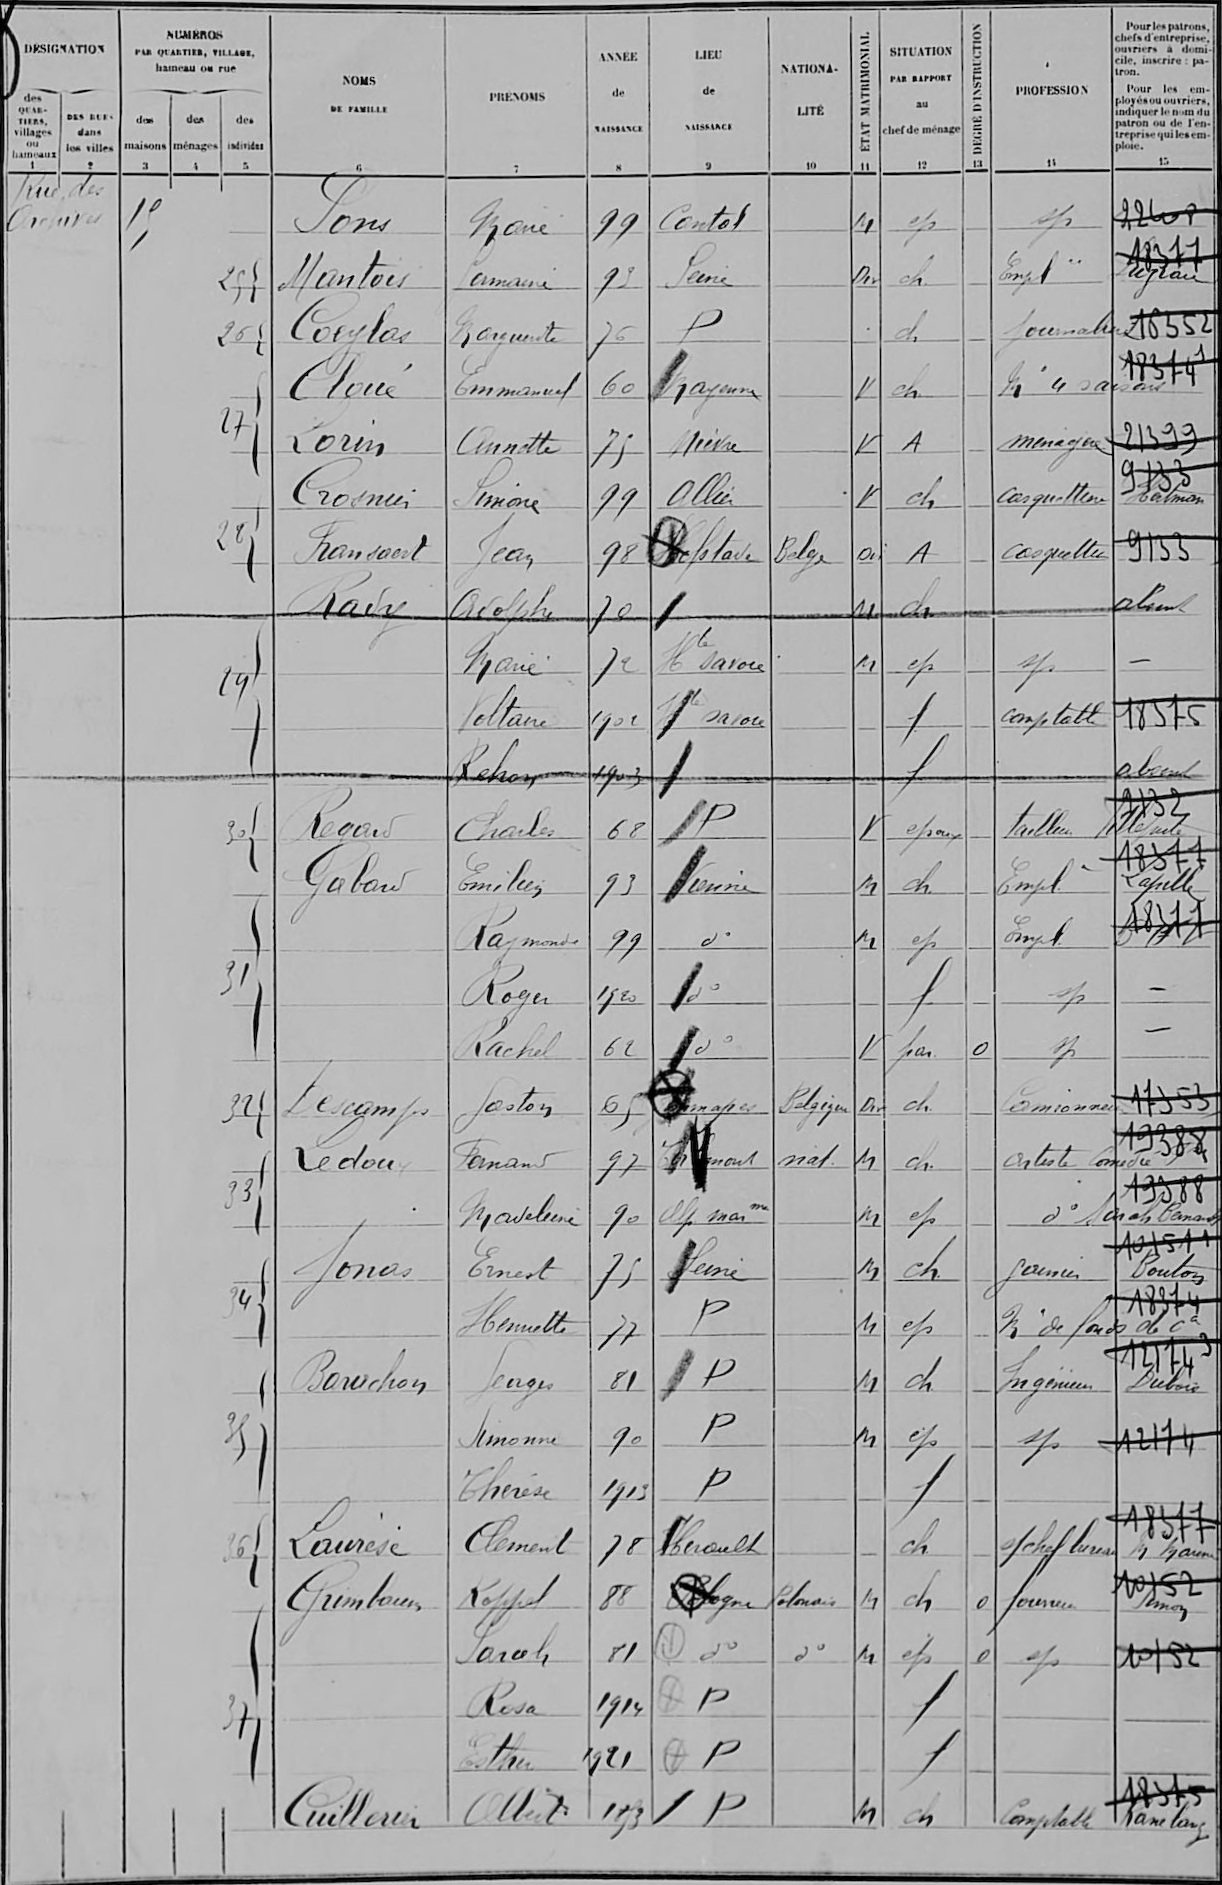Lons/Marie/99/Cantal/¤/M/ep/¤/sp/22408
Mantois/Germaine/93/Seine/¤/Div/ch /¤/Emplee/Aiglan?18377
Coeylas/Marguerite/76/P/¤/¤/ch/¤/Journalière/16352
Cloué/Emmanuel/60/Mayenne/¤/V/Ch/¤/Me 4 saisons/?18374
Lorin/Annette/75/Nièvre/¤/V/A/¤/ménagère/21399
Crosnier/Simone/99/Allier/¤/V/ch/¤/casquetteur/Hatman?9133
Fransaert/Jean/98/Hefstave/Belge/Div/A/¤/casquettier/9133
Rady/Adolphe/70/¤/¤/M/ch/¤/¤/absent
¤/Marie/72/Hte savoie/¤/M/ep/¤/sp/¤
¤/Voltaire/1902/Hte savoie/¤/¤/f/¤/comptable/18375
¤/Rehon/1903/¤/¤/¤/f/¤/¤/absent
Regard/Charles/68/P/¤/V/époux/¤/tailleur/Lillefaite?9132
Gabard/Emilien/93/Vienne/¤/M/ch /¤/Emplé/Lapelle?18377
¤/Raymonde/99/d°/¤/M/ep/¤/Empl /BHL?18377
¤/Roger/1920/d°/¤/¤/f/¤/sp/¤
¤/Rachel/62/d°/¤/V/par/0/p/¤
Lescamps/Gaston/65/Jemapes/Belgique/Div/ch /¤/Camionneur/17353
Ledoux/Fernand/97/C du nord/nat /M/ch /¤/artiste Comédie/?19388
¤/Madeleine/90/Alp Marme/¤/M/ep/¤/d°/Sarah Bernard?19388
Jonas/Ernest/75/Seine/¤/M/ch/¤/gainier/Bouton?10151
¤/Henriette/77/P/¤/M/ep/¤/me de fonds de Cce/?18374
Baruchon/Georges/81/P/¤/M/ch/¤/Ingénieur/Dubois?12174
¤/Simonne/90/P/¤/M/ep/¤/sp/12174
¤/Thérèse/1913/P/¤/¤/f/¤/¤/¤
Laurèse/Clément/78/Hérault/¤/¤/ch/¤/O- chef bureau de mairie/?18377
Grimbaun/Koppel/88/Pologne/Polonais/M/ch/0/fourreur/Simon?10152
¤/Sarah/81/d°/d°/M/ep/0/sp/10152
¤/Rosa/1914/P/¤/¤/f/¤/¤/¤
¤/Esther/1921/P/¤/¤/f/¤/¤/¤
Cuillerier/Albert/1853/P/¤/M/ch/¤/Comptable/Ranelang?18375
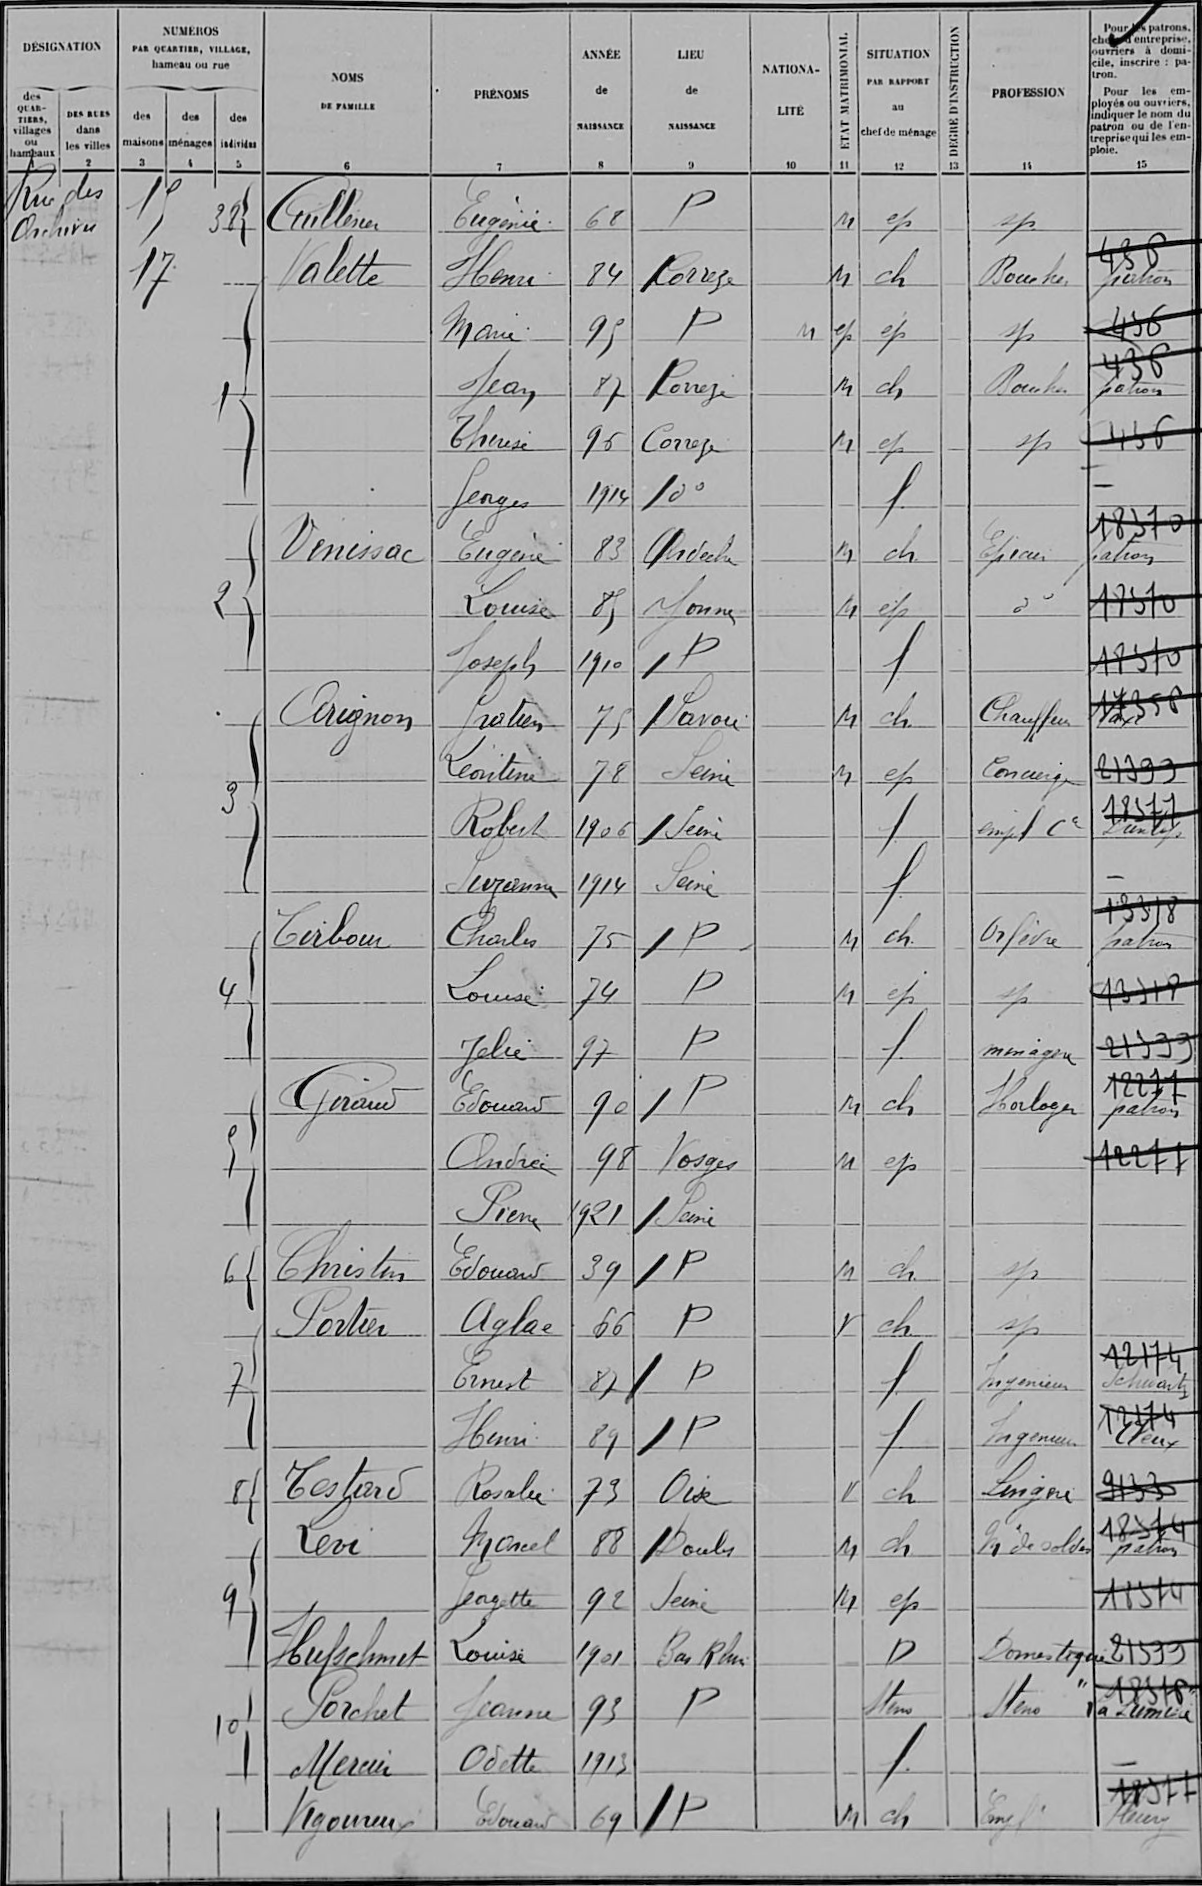Cuillener/Eugénie/68/P/¤/M/ep/¤/sp/¤
Valette/Henri/84/Correze/¤/M/ch/¤/Boucher/patron?436
¤/Marie/95/P/¤/M/ep/¤/sp/436
¤/Jean/87/Correze/¤/M/ch/¤/Boucher/patron?436
¤/Therese/95/Correze/¤/M/ep/¤/sp/436
¤/Georges/1914/d°/¤/¤/f/¤/¤/¤
Venissac/Eugène/83/Ardèche/¤/M/ch/¤/Epicier/patron?18370
¤/Louise/85/Yonne/¤/M/ep/¤/d°/18370
¤/Joseph/1910/P/¤/¤/f/¤/¤/18370
Arignon/Gratien/75/Savoie/¤/M/ch/¤/Chauffeur taxi/?17356
¤/Léontine/78/Seine/¤/M/ep/¤/Concierge/21399
¤/Robert/1906/Seine/¤/¤/f/¤/empl Cce/Dunlop?18377
¤/Suzanne/1914/Seine/¤/¤/f/¤/¤/¤
Tirbour/Charles/75/P/¤/M/ch/¤/Orfèvre/patron?13318
¤/Louise/74/P/¤/M/ep/¤/sp/13318
¤/Zelie/97/P/¤/¤/f/¤/ménagère/21399
Giraud/Edouard/90/P/¤/M/ch/¤/Horloger/patron?12277
¤/Andrée/98/Vosges/¤/M/ep/¤/¤/12277
¤/Pierre/1921/Seine/¤/¤/¤/¤/¤/¤
Christin/Edouard/39/P/¤/M/ch/¤/sp/¤
Portier/Aglae/66/P/¤/V/ch/¤/sp/¤
¤/Ernest/87/P/¤/¤/f/¤/Ingénieur/Schwartz?12174
¤/Henri/89/P/¤/¤/f/¤/Ingénieur/Cleux?12174
Testard/Rosalie/73/Oise/¤/V/ch/¤/Lingère/9133
Levi/Marcel/88/Doubs/¤/M/ch/¤/M de soldes/patron?18374
¤/Georgette/92/Seine/¤/M/ep/¤/¤/18374
Hufschmit/Louise/1901/Bas Rhin/¤/¤/D/¤/Domestique/21399
Porchet/Jeanne/93/P/¤/¤/Steno/¤/Steno/La lumiere?18376
Mercier/Odette/1913/¤/¤/¤/f /¤/¤/¤
Vigoureux/Edouard/69/P/¤/M/ch/¤/Empl/Henry?18377